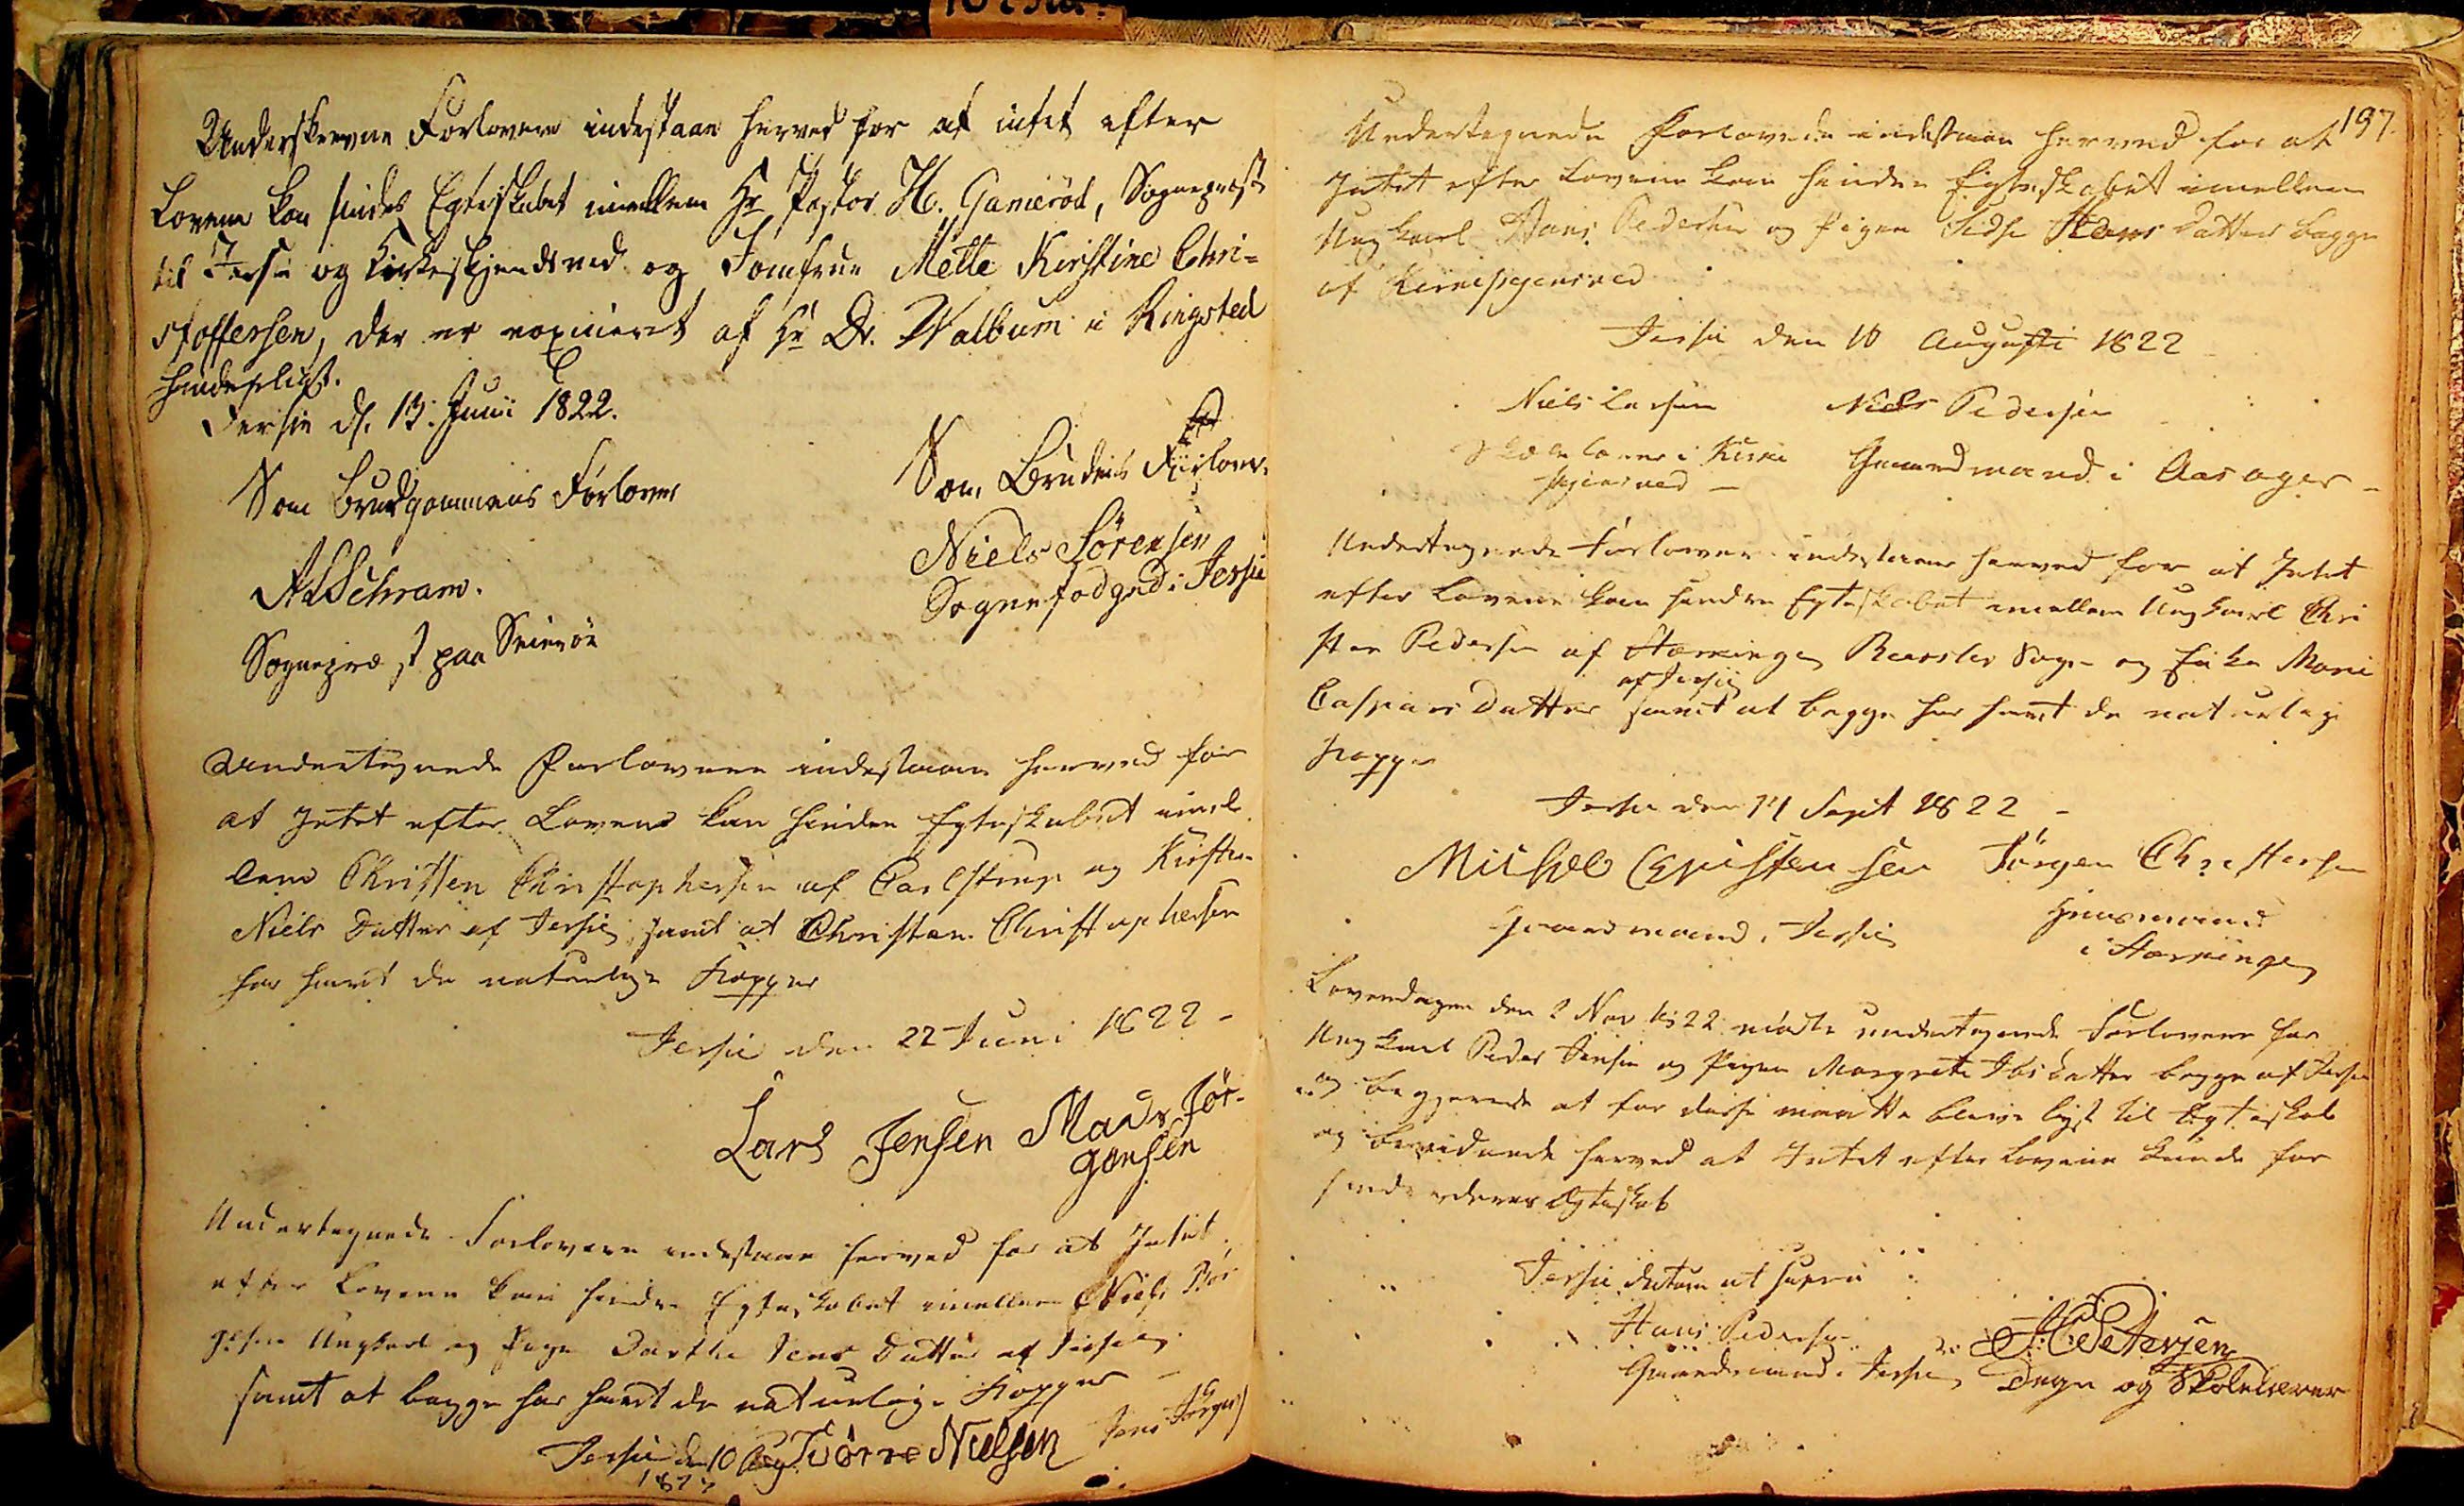Underskrevne Forlovere indestaar herved for at intet efter
Lovene kan hindre Egteskabet imellem Hr Pastor H. Gamerød, Sognepræst
til Jersie og Kirkeskjendsved og Jomfrue Mette Kirstine Chri¬
stoffersen, der er vaxineret af Hr Dr. Walbum i Ringsted
hinderligt.##
Jersie d. 13. Junii 1822.
Som Brudgommens Forlover
A L Schram.
Sognepræst paa Seierøe
Som Brudens Forlover
Niels Sørensen
Sognefodged i Jersie
Undertegnede Forlovere indestaar herved for
at Intet efter Lovene kan hindre Egteskabet imel¬
lem Christen Christophersen af Carlstrup og Kirsten
Niels Datter af Jersie, samt at Christen Christophersen
har havt de naturlige Kopper
Jersie den 22 Juni 1822
Lars Jensen  Mads Jør¬
gensen
Undertegnede Forlovere indestaar herved for at Intet
efter Lovene kan hindre Egteskabet imellem Niels Bør
gesen Ungkarl og Pige Dorthe Jens Datter af Jersie,
samt at begge har havt de naturlige Kopper -
Jersie den 10 Aug Børre Nielsen Jens Jørgens
1822
197.
Undertegnede Forlovere indestaar herved for at
Intet efter Lovene kan hindre Egteskabet imellem
Ungkarl Hans Pedersen og Pigen Sidse Hans Datter begge
af Kirkeskjensved.
Jersie den 10 Augusti 1822
Niels Larsen
Niels Pedersen
Skolelærer i Kirke
Gaardmand i Aasager
skjensved   
Undertegnede Forlovere indestaar herved for at Intet
efter Lovene kan hindre Egteskabet imellem Ungkarl Chri
sten Pedersen af Stærkinge Reerslev Sogn. og Enke Marie
af Jersie
Caspars Datter samt at begge har havt de naturlige
Kopper
Jersie den 14 Sept 1822 -
Michel Christensen Jørgen Christensen
Huusmand
Gaardmand i Jersie
i Stærkinge
Løverdagen den 2 Nov 1822 mødte undertegnede Forlovere for
Ungkarl Peder Jensen og Pigen Margrete IbsDatter begge af Jersie
og begjerede at for disse maatte blive lyst til Egteskab
og bevidnede herved at Intet efter Lovene kunde for
hindre deres Ægteskab
Jersie Datum ut supra
Hans Pedersen
H: Petersen
Gaardmand i Jersie Degn og Skolelærer
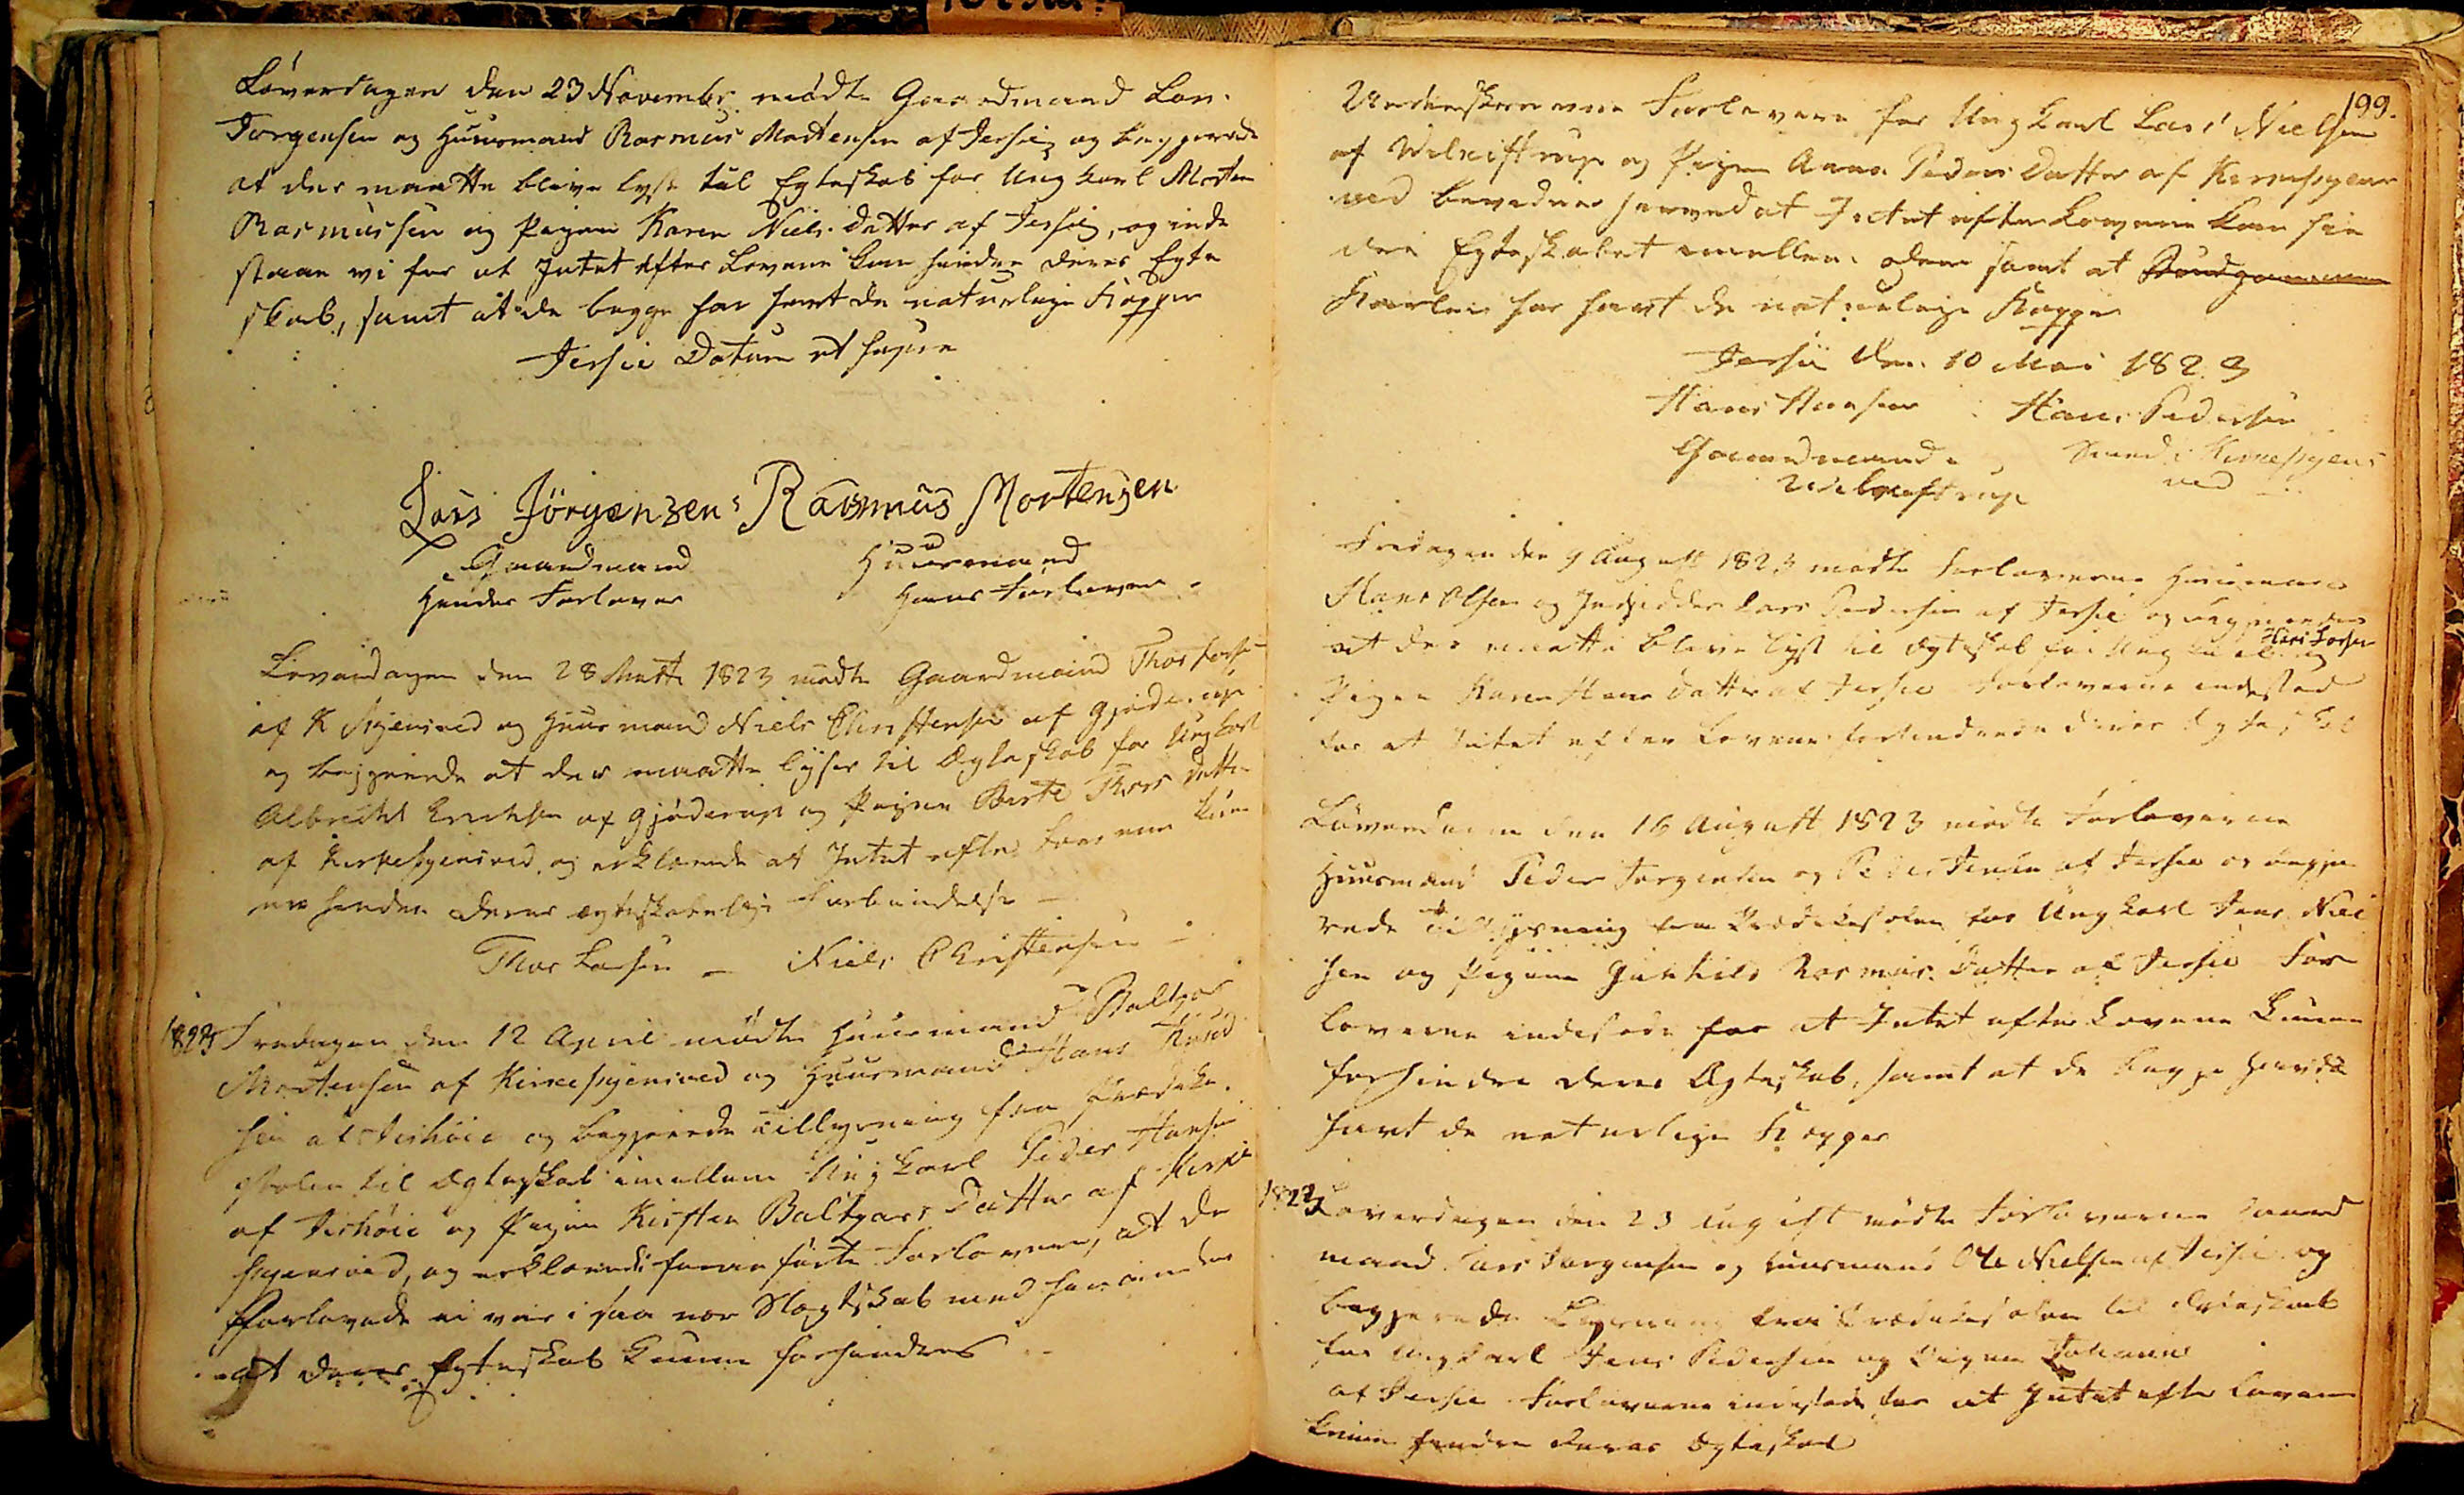Løverdagen den 23 Novembr mødte Gaardmand Lars.
Jørgensen og Huusmand Rasmus Mortensen af Jersie og bejgerede
at der maatte blive lyst til Egteskab for Ungkarl Morten
Rasmussen og Pigen Karen Niels. Datter af Jersie, og inde
staae vi for at Intet efter Loven kan hindre deres Egte
skab, samt at de begge har havt de naturlige Kopper
Jersie Datum ut Supra
Lars Jørgensen Rasmus Mortensen
Huusmand
Gaardmand
Hans Forlover.
Hendes Forlover
Løverdagen den 28 Mart 1823 mødte Gaardmand Thor Larsen
af K Skjensved og Huusmand Niels Christensen af Gjørderup
og bejgerede at der maatte lyses til Ægteskab for Ungkarl
Albrecht Erichsen af Gjørderup og Pigen Birte Thors Datter
af Kirkeskjensved, og erklærede at Intet efter Lovene kun
ne hindre deres ægteskabelige forbindelse
Thor Larsen
Niels Christensen
Fredagen den 12 April mødte Huusmand Baltzar
Mortensen af Kirkeskjensved og Huusmand Hans Knud
sen af Iishøie og begjerede Tillysning fra Prædike¬
stolen til Ægteskab imellem Ungkarl Peder Hansen
af Iishøie og Pigen Kirsten Baltzars Datter af Kirke
skjensved, og erklærede foran førte Forlovere, at de
Forlovede ei var i saa nær Slægtskab med hinanden
at deres Egteskab kunne forhindres. 
199.
Underskrevne var Forlovere for Ungkarl Lars Nielsen
af Wilkistrup og Pigen Anne Peders Datter af Kirkeskjens
ved bevidner herved at Intet efter Lovene kan hin
dre Egteskabet imellem dem samt at Brudgommen
Karlen har havt de naturlige Kopper
Jersie den 10 Mai 1823
Hans Hansen
Hans Pedersen
Gaardmand i
Smed i Kirkeskjens
ved
Vilkestrup
Fredagen den 9 August 1823 mødte Forloverne Huusmand
Hans Olsen og Indsidder Lars Pedersen af Jersie og begjerede
Hans Larsen
at der maatte blive lyst til Ægteskab for Ungkarl og
Pigen Karen Hans Datter af Jersie. Forloverne indestod
for at Intet efter Lovene forhindrede deres Ægteskab
Løverdagen den 16 August 1823 mødte Forloverne
Huusmand Peder Jørgensen og Peder Jensen af Jersie og begje
rede Tillysning fra Prædikestolen for Ungkarl Jens Niel
sen og Pigen Gunhild Rasmus Datter af Jersie - For
loverne indestode for at Intet efter Lovene kunne
forhindre deres Ægteskab, samt at de begge havde
havt de naturlige Kopper
1823 Løverdagen den 23 August mødte Forloverne Gaard
mand Lars Jørgensen og Huusmand Ole Nielsen af Jersie og
begjerede Lysning fra Prædikestolen til Ægteskab
for Ungkarl Jens Pedersen og Pigen Johanne
af Jersie Forloverne indestode for at Intet efter Lovene
kunne hindre deres Ægteskab